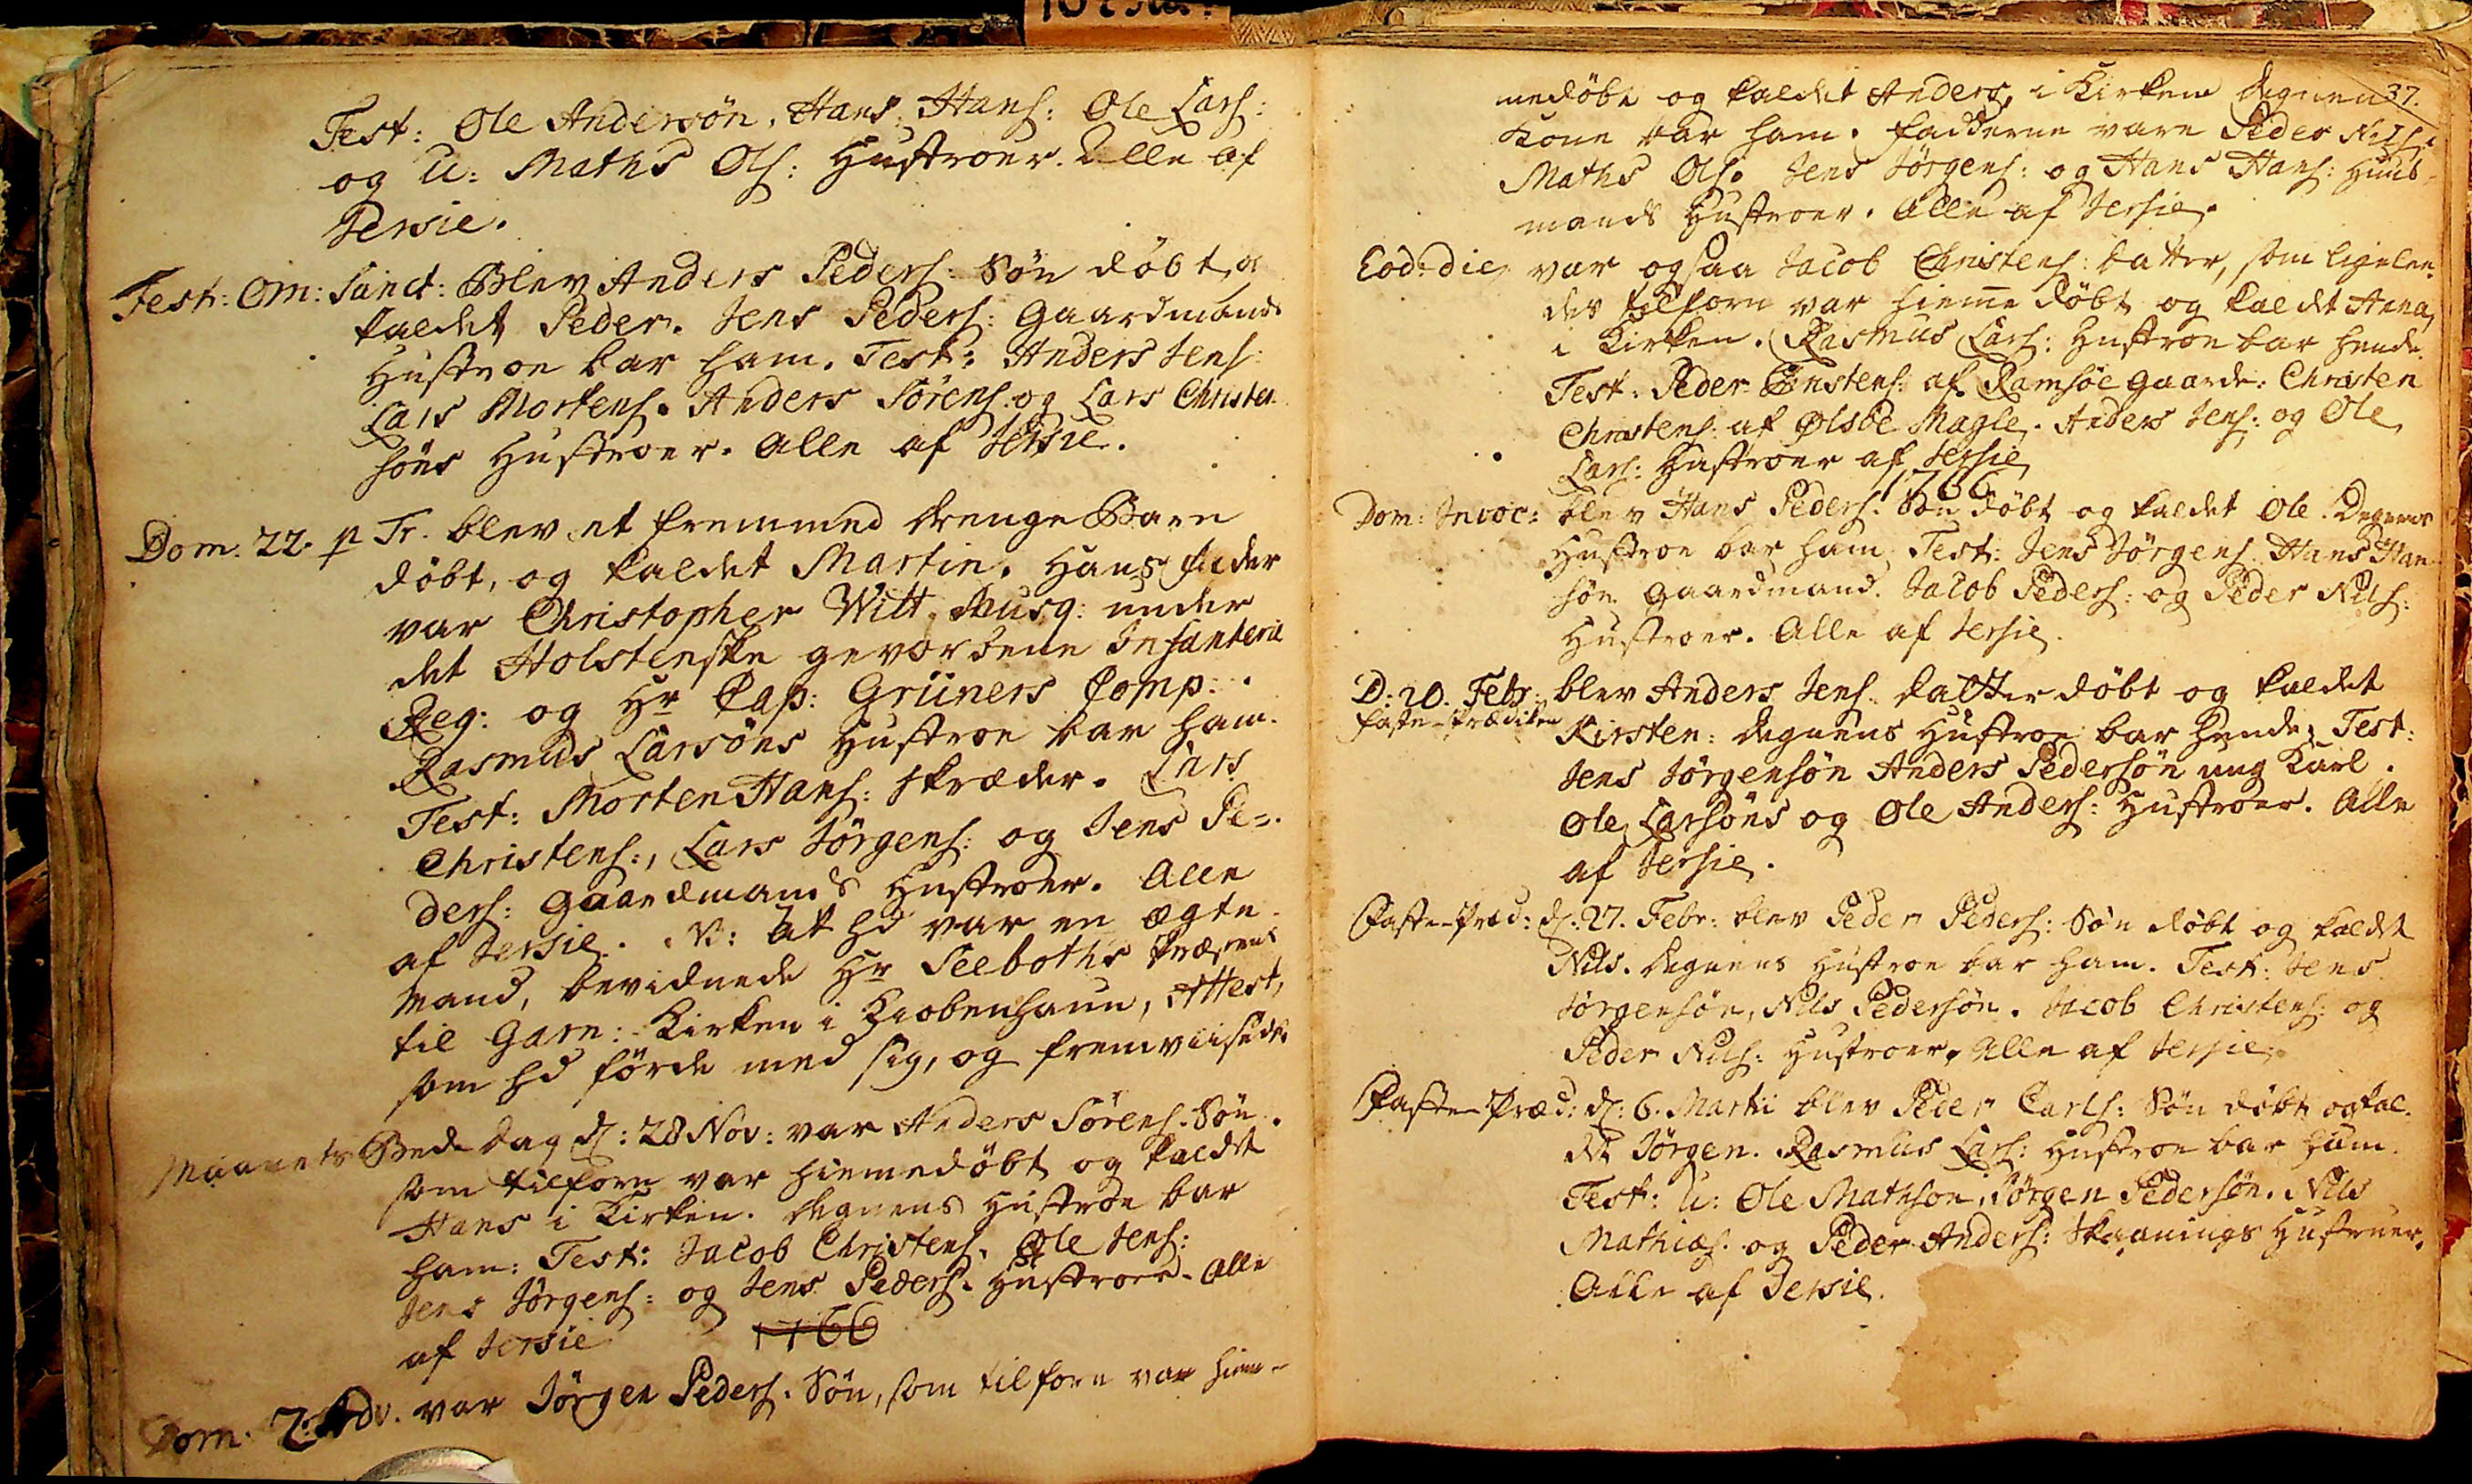Test: Ole Andersøn, Hans Hans: Ole Lars:
og U: Maths Ols: Hustroer. Alle af
Jersie.
Fest: Om: Sanct: blev Anders Peders: Søn døbt og
kaldet Peder. Jens Peders: Gaardmands
Hustroe bar ham. Test: Anders Jens:
Lars Mortens. Anders Sørens. og Lars Christen
søns Hustroer. Alle af Jersie.
Dom: 22. p Tr. blev et fremmed Drenge Barn
døbt, og kaldet Martin. Hans Fader
var Christopher Witt, Musq: under
det Holstenske gevorbene Infanterie
Reg: og Hr Cap Grüners Comp:
Rasmus Larsøns Hustroe bar ham.
Test: Morten Hans: Skræder. Lars
Christens:, Lars Jørgens: og Jens Pe¬
ders: Gaardmands Hustroer. Alle 
af Jersie. NB: at hd var en ægte
mand, bevidnede Hr Seeboths Præsten
til Garn: Kirken i Kiøbenhaun, Attest
som hd førde med sig, og fremviisede
Maanets Bede Dag d: 28 Nov: var Anders Sørens. Søn.
som tilforn var hiemmedøbt og kaldt
Hans i Kirken. Degnens Hustroe bar 
ham: Test: Jacob Christens. Ole Jens:
Jens Jørgens: og Jens Peders. Hustroer. Alle
af Jersie
1766 
Dom: 2: Adv. var Jørgen Peders: Søn, som tilforn var hiem¬
37.
medøbt og kaldet Anders, i Kirken Degnens
Kone bar ham. Fadderne vare Peder Nils.,
Maths Ols. Jens Jørgens: og Hans Hans: Huus¬
mands Hustroer. Alle af Jersie
Eod: die var ogsaa Jacob Christens: Datter, som ligelee¬
des tilforn var hiemmedøbt og kaldt Anna,
i Kirken. Rasmus Lars: Hustroe bar hende.
Test: Peder Christens: af Ramsøe Gaard: Christen
Christens: af Ølsøe Magle. Anders Jens: og Ole
Lars: Hustroer af Jersie.
1766
Dom: Invoc: blev Hans Peders. Søn døbt og kaldet Ole. Degnens
Hustroe bar ham. Test: Jens Jørgens. Hans Han¬
søn Gaardmand. Jacob Peders: og Peder Nils:
Hustroer. Alle af Jersie.
D: 20. Febr: blev Anders Jens. Datter døbt og kaldet
Faste-Prædiken
Kirsten: Degnens Hustroe bar hende. Test:
Jens Jørgensøn Anders Pedersøn ungkarl.
Ole Larsøns og Ole Anders: Hustroer. Alle
af Jersie.
Faste-Præd: d: 27 Febr: blev Peder Peders: Søn døbt og kaldt
Nils. Degnens Hustroe bar ham. Test: Jens 
Jørgensøn, Nils Pedersøn. Jacob Christens: og
Peder Nils: Hustroer. Alle af Jersie.
Faste-Præd: d: 6. Martii blev Peder Carls: Søn døbt og kal¬
dt Jørgen. Rasmus Lars: Hustroe bar ham.
Test: U: Ole Mathsøn, Jørgen Pedersøn. Nils
Mathiæs. og Peder Anders: Skaanings Hustroer.
Alle af Jersie.
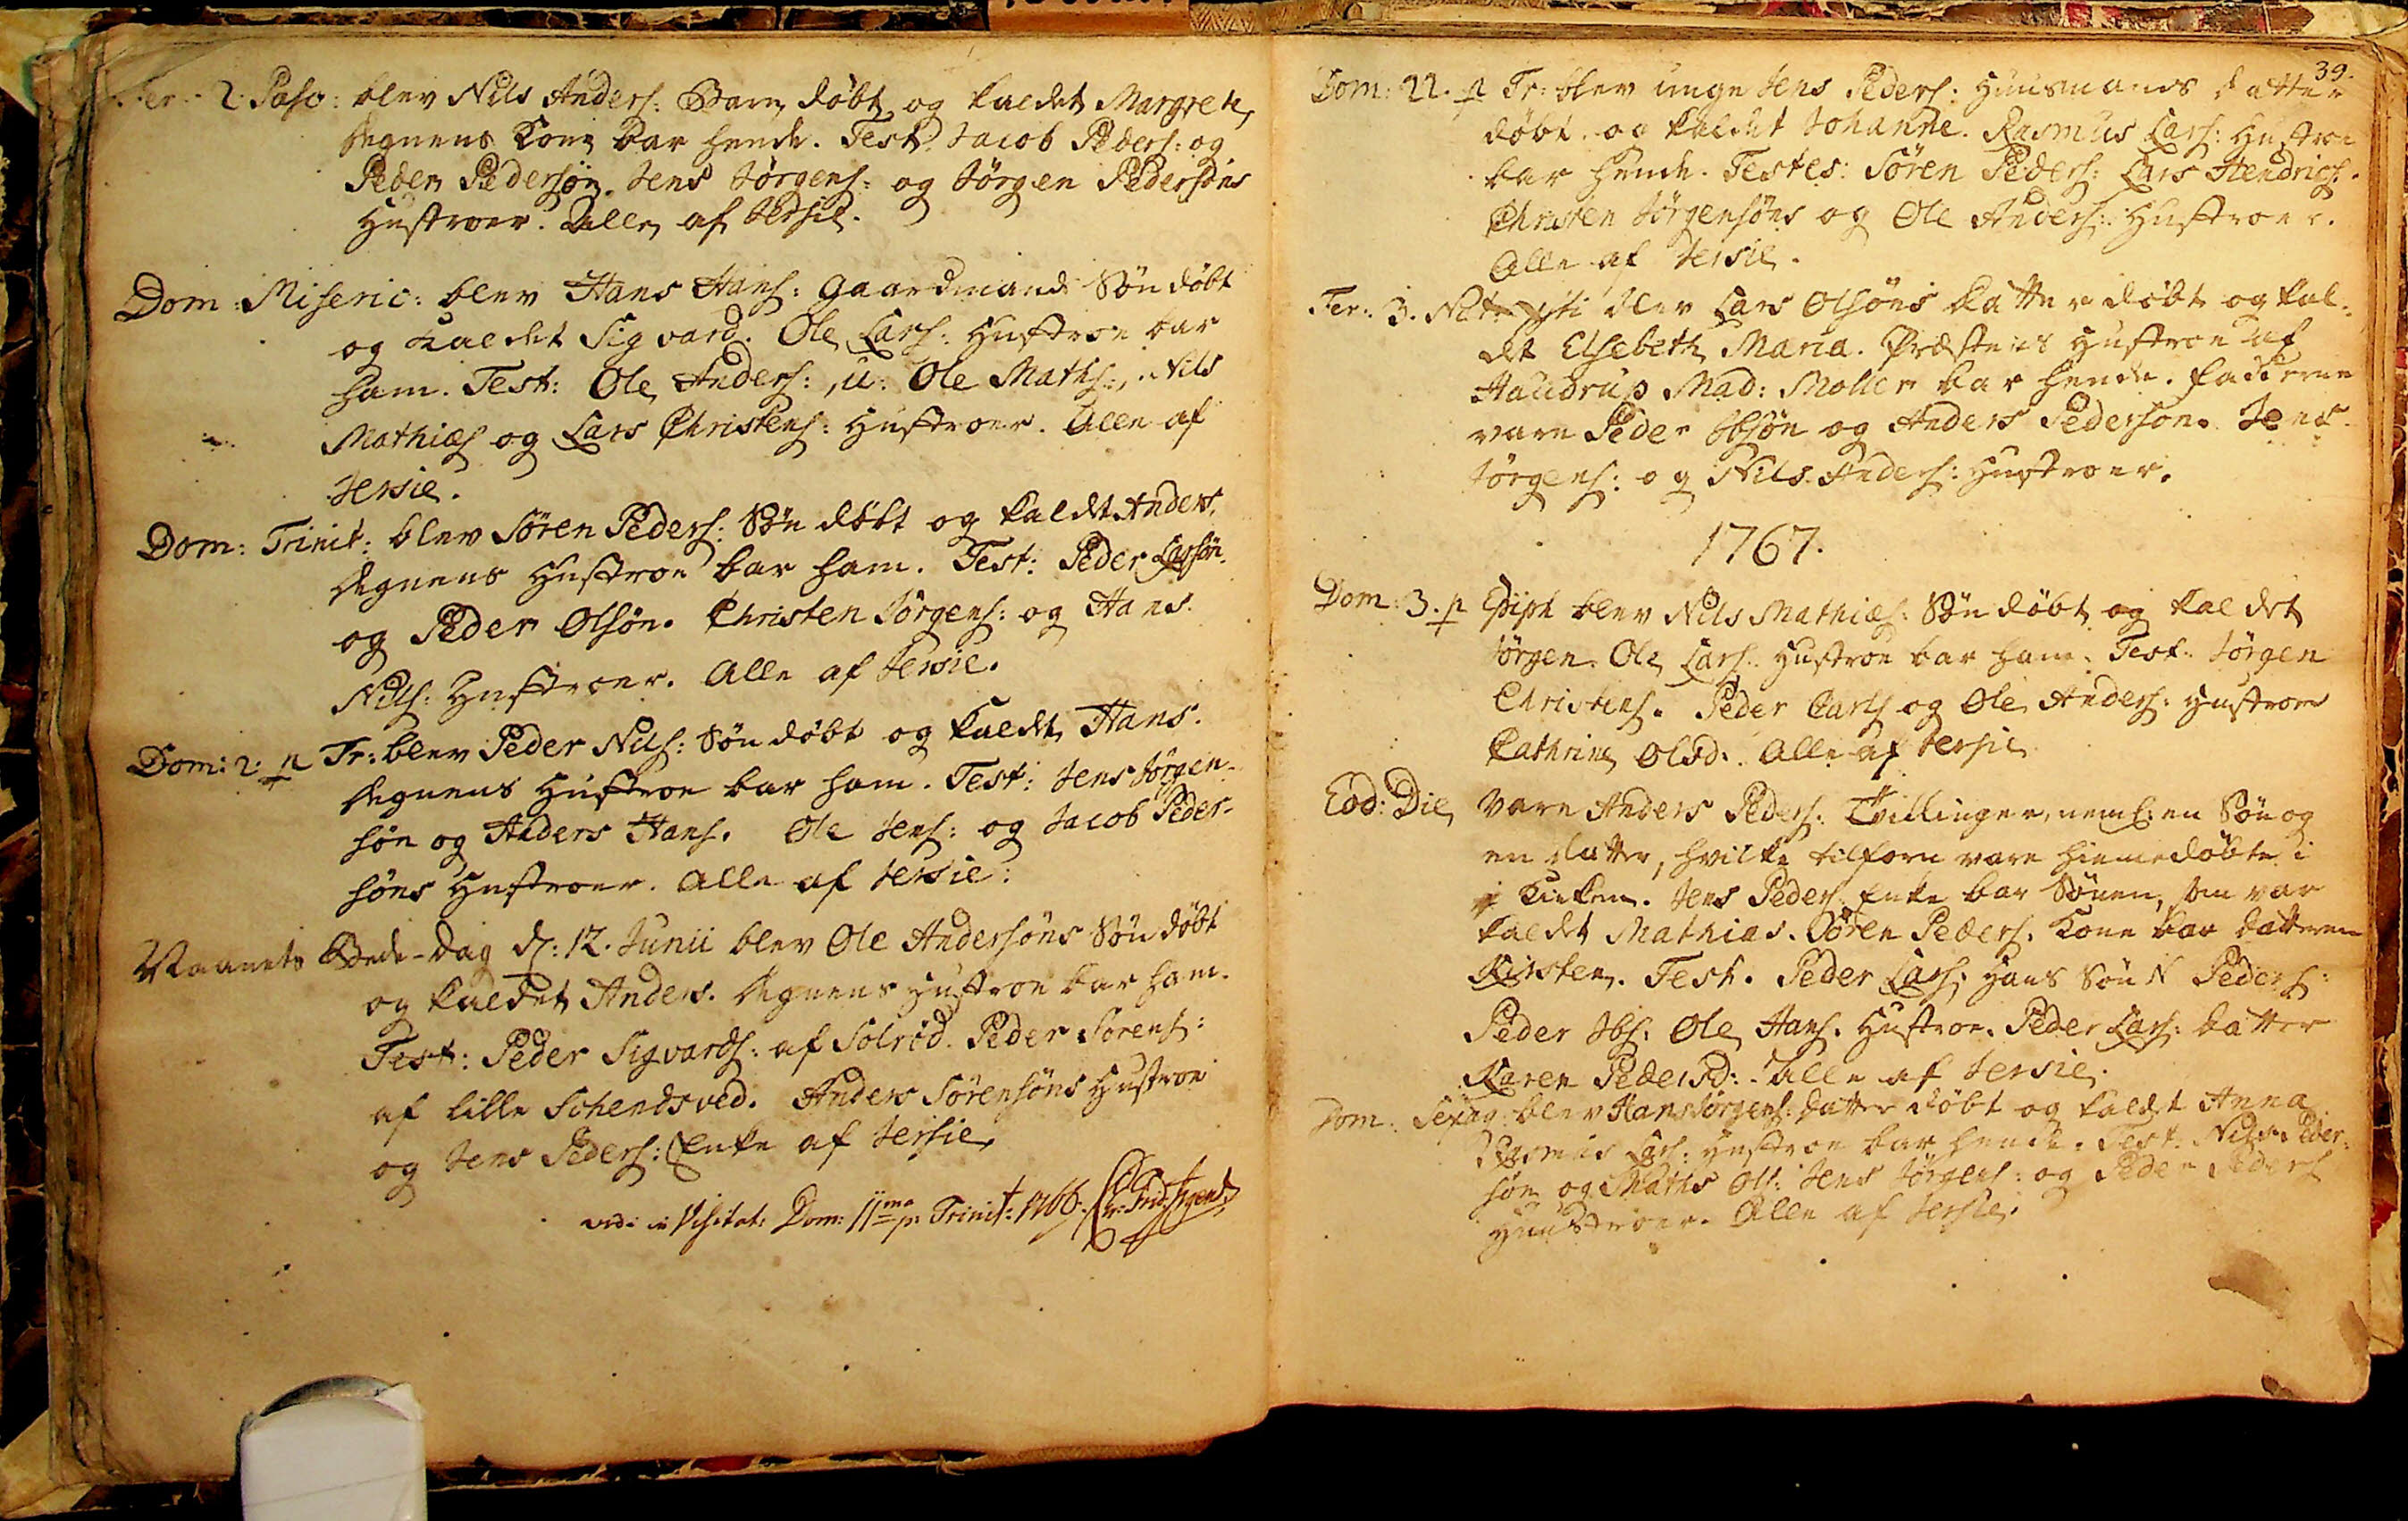Fer. 2. Pasc: blev Nils Anders: Barn døbt og kaldet Margrete.
Degnens Kone bar hende. Test. Jacob Peders: og
Peder Pedersøn. Jens Jørgens: og Jørgen Pedersøns
Hustroer. Alle af Jersie.
Dom: Miseric: blev Hans Hans: Gaardmand Søn døbt
og kaldet Sigvard. Ole Lars: Hustroe bar
ham. Test: Ole Anders:, U: Ole Maths:, Nils
Mathiæs og Lars Christens: Hustroer. Alle af
Jersie.
Dom: Trinit: blev Søren Peders: Søn døbt og kaldet Anders.
Degnens Hustroe bar ham. Test: Peder Larsøn
og Peder Olsøn. Christen Jørgens: og Hans
Nils. Hustroer. Alle af Jersie.
Dom: 2: p Tr: blev Peder Nils: Søn døbt og kaldt Hans:
Degnens Hustroe bar ham. Test: Jens Jørgen¬
søn og Anders Hans. Ole Jens: og Jacob Peder¬
søns Hustroer. Alle af Jersie.
Maanets Bede-Dag d: 12. Junii blev Ole Andersøns Søn døbt
og kaldet Anders. Degnens Hustroe bar ham.
Test: Peder Sigvards: af Solrød. Peder Sørens:
af Lille Schendsved. Anders Sørensøns Hustroe
og Jens Peders: Enke af Jersie.
vidi i Visitat: Dom: 11mo p: Trinit: 1766: Chr: Fried: Irgens
39.
Dom: 22. p Tr: blev unge Jens Peders: Huusmands datter
døbt og kaldet Johanne. Rasmus Lars: Hustroe
bar hende. Testes: Søren Peders: Lars Hendrics:
Christen Jørgensøns og Ole Anders: Hustroer.
Alle af Jersie
Fer: 3. Nat: Xsti blev Lars Olsøns Datter døbt og kal¬
dt Elsebeth Maria. Præstens Hustroe af 
Haudrup Mad: Moller bar hende. Fadderne
vare Peder Ibsøn og Anders Pedersøn. Jens 
Jørgens: og Nils Anders: Hustroer.
1767.
Dom: 3. p Epiph blev Niels Mathiæs: Søn døbt og kaldet
Jørgen. Ole Lars. Hustroe bar ham. Test: Jørgen
Christens. Peder Carls og Ole Anders. Hustroer
Cathrine Olsd: Alle af Jersie.
Eod: Die vare Anders Peders: Tvillinger, neml: en Søn og
en datter, hvilke tilforn vare hiemmedøbte, i
i Kirken. Jens Peders: Enke bar Sønen, som var 
kaldt Mathias. Søren Peders: Kone bar Datteren
Kirsten. Test. Peder Lars. Hans Søn N Peders:
Peder Ibs: Ole Hans: Hustroe. Peder Lars: Datter
Karen Pedersd:. Alle af Jersie.
Dom: Sexag: blev Hans Jørgens: Datter døbt og kaldet Anna.
Rasmus Lars: Hustroe bar hende: Test: Nils Peder¬
søn og Maths Ols: Jens Jørgens: og Peder Peders:
Huustroer. Alle af Jersie.